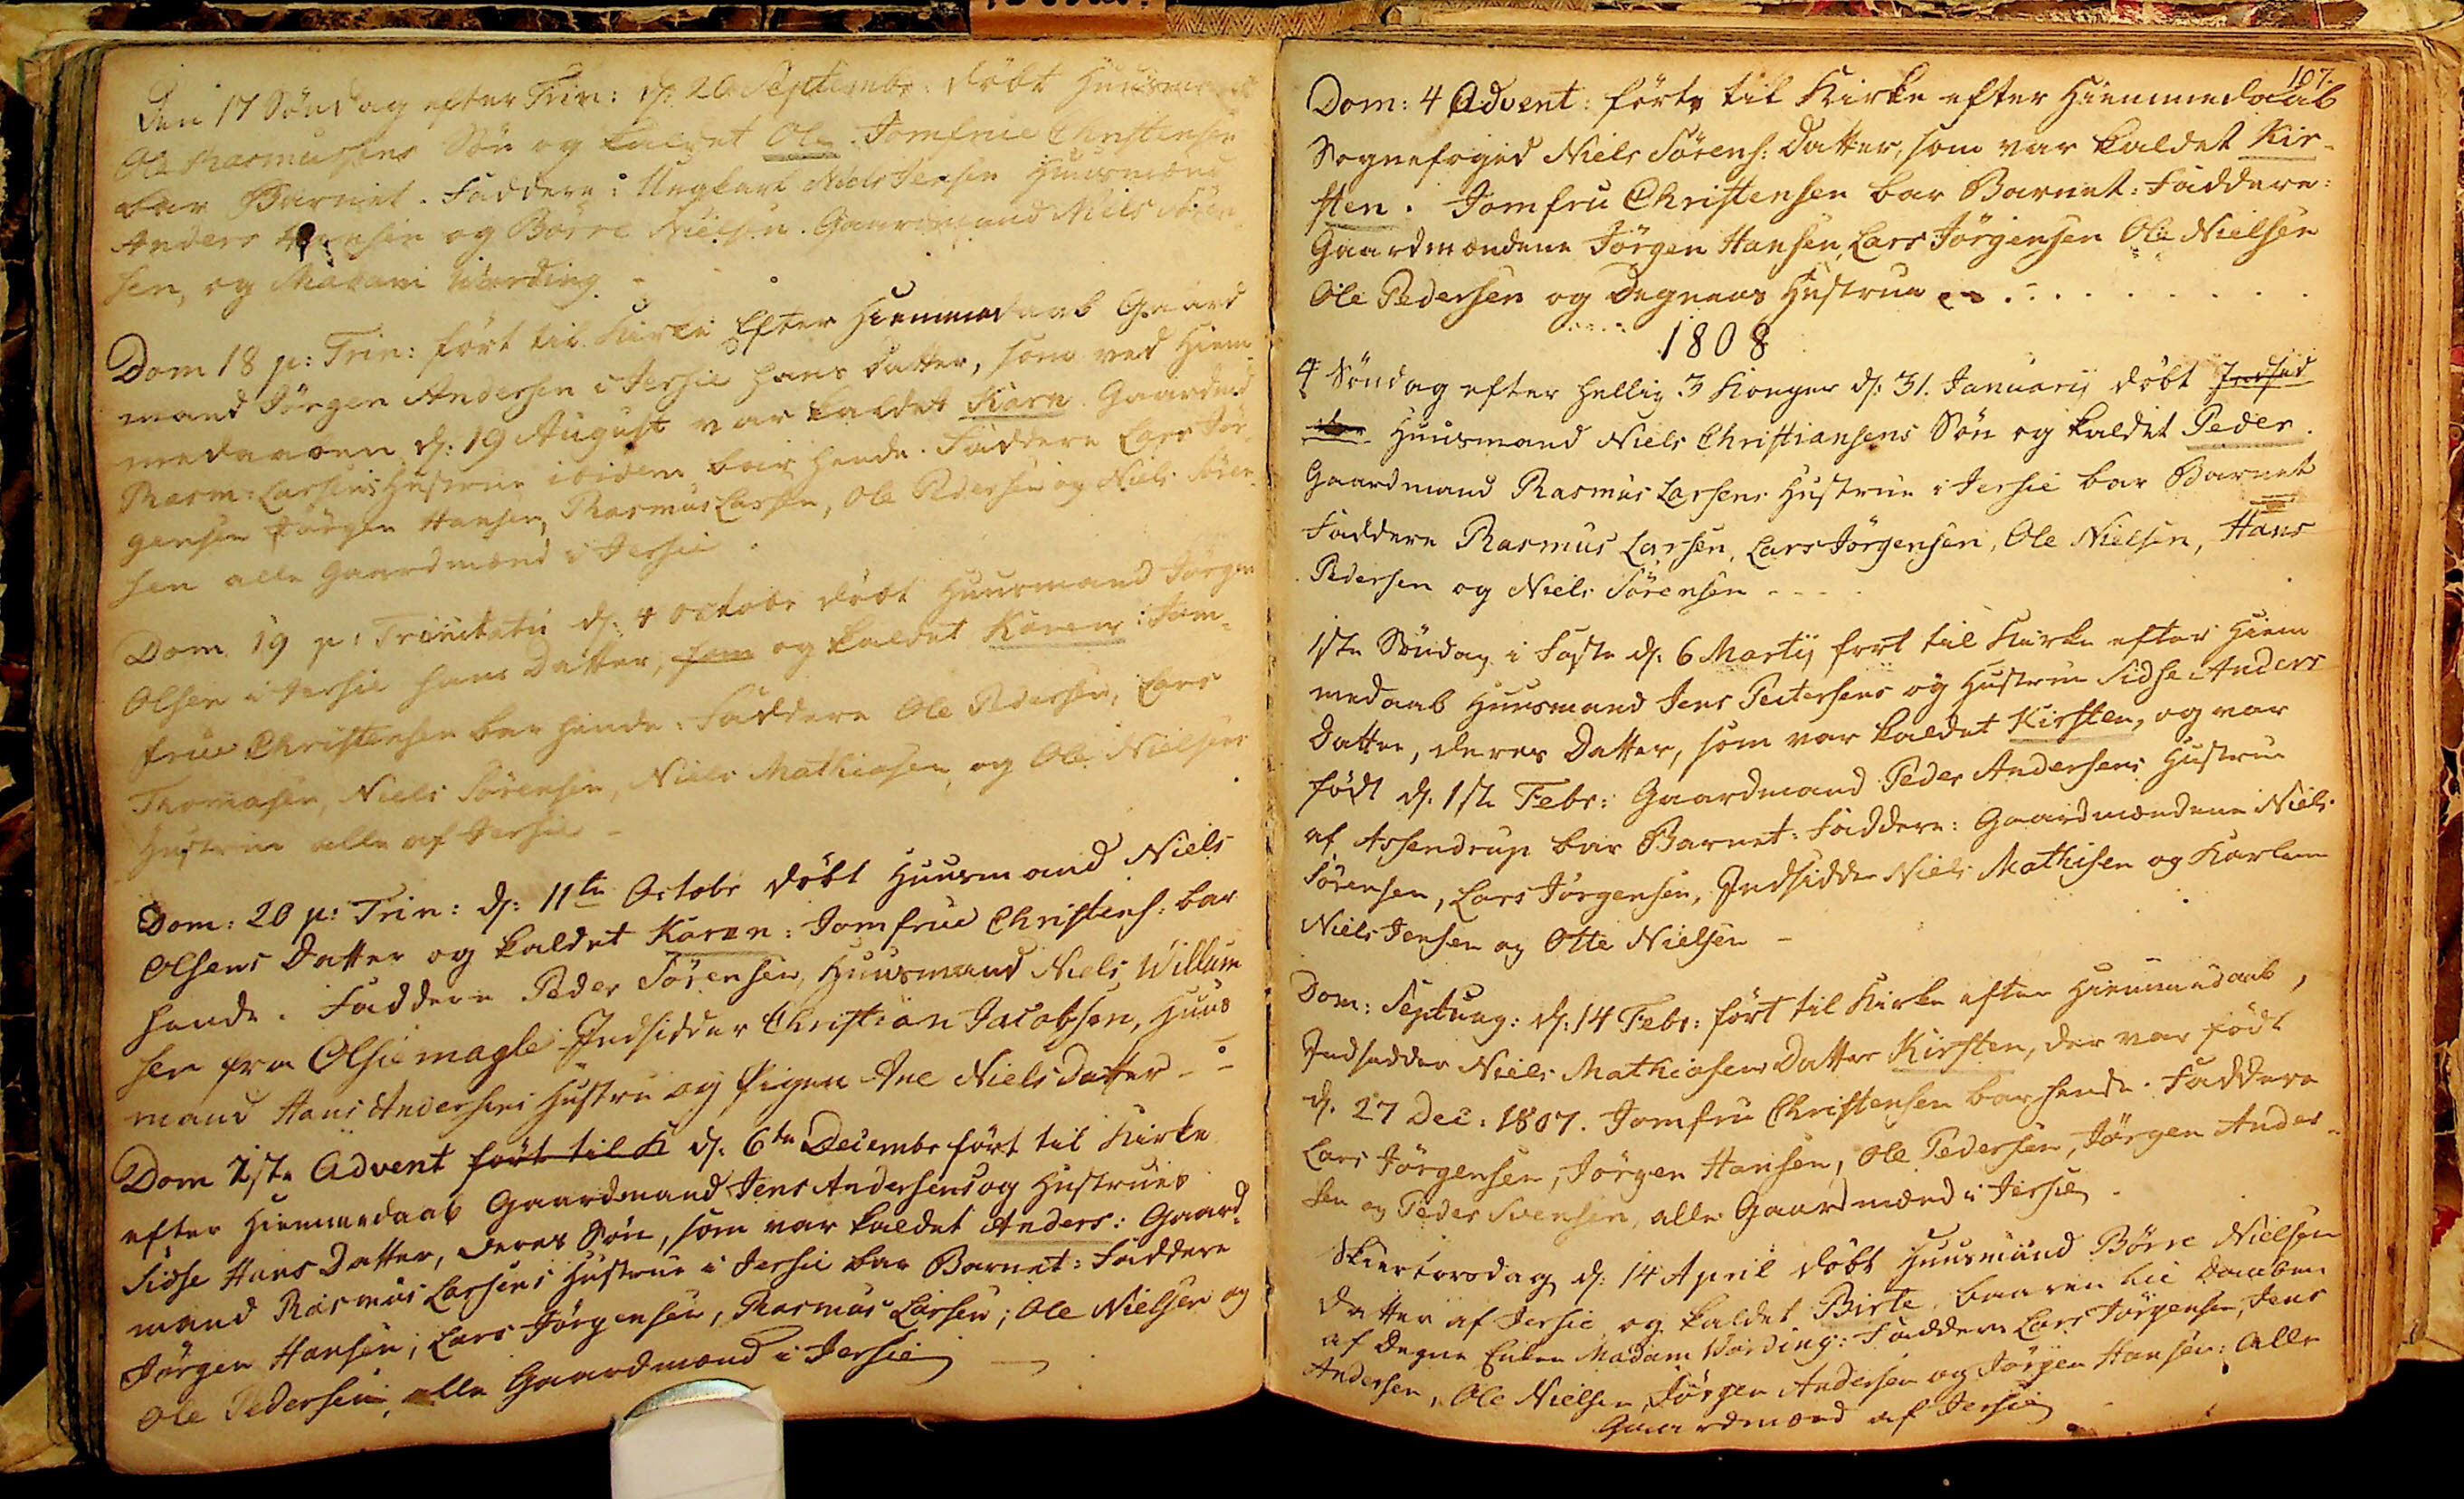Den 17 Søndag efter Trin: d: 20 Septembr: døbt Huusmand
Ole Rasmussens Søn og kaldet Ole. Jomfrue Christensen
bar Barnet. Faddere: Ungkarl Niels Jensen Huusmænd
Anders Hansen og Børre Nielsen. Gaardmand Niels Søren
sen, og Madam Wording.
Dom 18 p: Trin: ført til Kirke Efter Hiemmedaab Gaard
mand Jørgen Andersen i Jersie hans Datter, som ved Hiem
medaaben d: 19 August var kaldet Karn. Gaardmd
Rasm: Larsens Hustrue ibidem bar hende. Faddere Lars Jør
gensen, Jørgen Hansen, Rasmus Larsen, Ole Pedersen og Niels Søren
sen alle Gaardmænd i Jersie.
Dom. 19 p: Trinitatis d: 4 Octobr døbt Huusmand Jørgen
Olsen i Jersie hans Datter, som og kaldet Karen: Jom¬
frue Christensen bar hende: Faddere Ole Pedersen, Lars
Thomasen, Niels Sørensen, Niels Mathiasen, og Ole Nielsens
Hustrue alle af Jersie.
Dom: 20 p: Trin: d: 11te Octobr døbt Huusmand Niels
Olsens Datter og kaldet Karen: Jomfrue Christens: bar
hende. Faddere Peder Sørensen, Huusmand Niels Willum
sen fra Ølsiemagle Indsidder Christian Jacobsen, Huus
mand Hans Andersens Hustru og Pigen Ane Niels Datter_ _
Dom 2ste Advent ført til K d: 6te Decembr ført til Kirke
efter Hiemmedaab Gaadmand Jens Andersens og Hustrues
Sidse Hans Datter, deres Søn, som var kaldet Anders: Gaard¬
mand Rasmus Larsens Hustrue i Jersie bar Barnet: Faddere
Jørgen Hansen, Lars Jørgensen, Rasmus Larsen; Ole Nielsen og
Ole Pedersen, alle Gaardmænd i Jersie
107.
Dom: 4 Advent: ført til Kirke efter Hiemmedaab
Sognefoged Niels Sørens: Datter, som var kaldet Kir¬
sten. Jomfru Christensen bar Barnet: Faddere:
Gardmændene Jørgen Hansen, Lars Jørgensen Ole Nielsen
Ole Pedersen og Degnens Hustrue
1808
4 Søndag efter hellig 3 Konger d: 31. Januarij døbt Indsid
der Huusmand Niels Christiansens Søn og kalet Peder.
Gaardmand Rasmus Larsens Hustrue i Jersie bar Barnet
Faddere Rasmus Larsen, Lars Jørgensen, Ole Nielsen, Hans
Pedersen og Niels Sørensen
1ste Søndag i Faste d: 6 Martij ført til Kirke efter Hiem
medaab Huusmand Jens Peitersens og hustrue Sidse Anders
Datter, deres Datter, som var kaldet Kirsten, og var
født d: 1ste Febr: Gaardmand Peder Andersens Hustrue
af Assendrup bar Barnet: Faddere: Gaardmændene Niels
Sørensen, Lars Jørgensen, Indsidder Niels Mathisen og karlene
Niels Jensen og Otte Nielsen.
Dom: Septuag: d: 14 Febr: ført til Kirke efter Hiemmedaab,
Indsidder Niels Mathiasens Datter Kirsten, der var født
d. 27 Dec: 1807. Jomfru Christensen bar hende. Faddere
Lars Jørgensen, Jørgen Hansen, Ole Pedersen, Jørgen Ander¬
sen og Peder Svensen, alle Gaardmænd i Jersie.
Skiertorsdag d: 14 April døbt Huusmand Børre Nielsens
Datter af Jersie og kaldet Birte, baaren til Daaben
af Degne Enken Madam Wording: Faddere: Lars Jørgensen, Jens
Andersen, Ole Nielsen, Jørgen Andersen og Jørgen Hansen: Alle
Gaardmænd af Jersie.
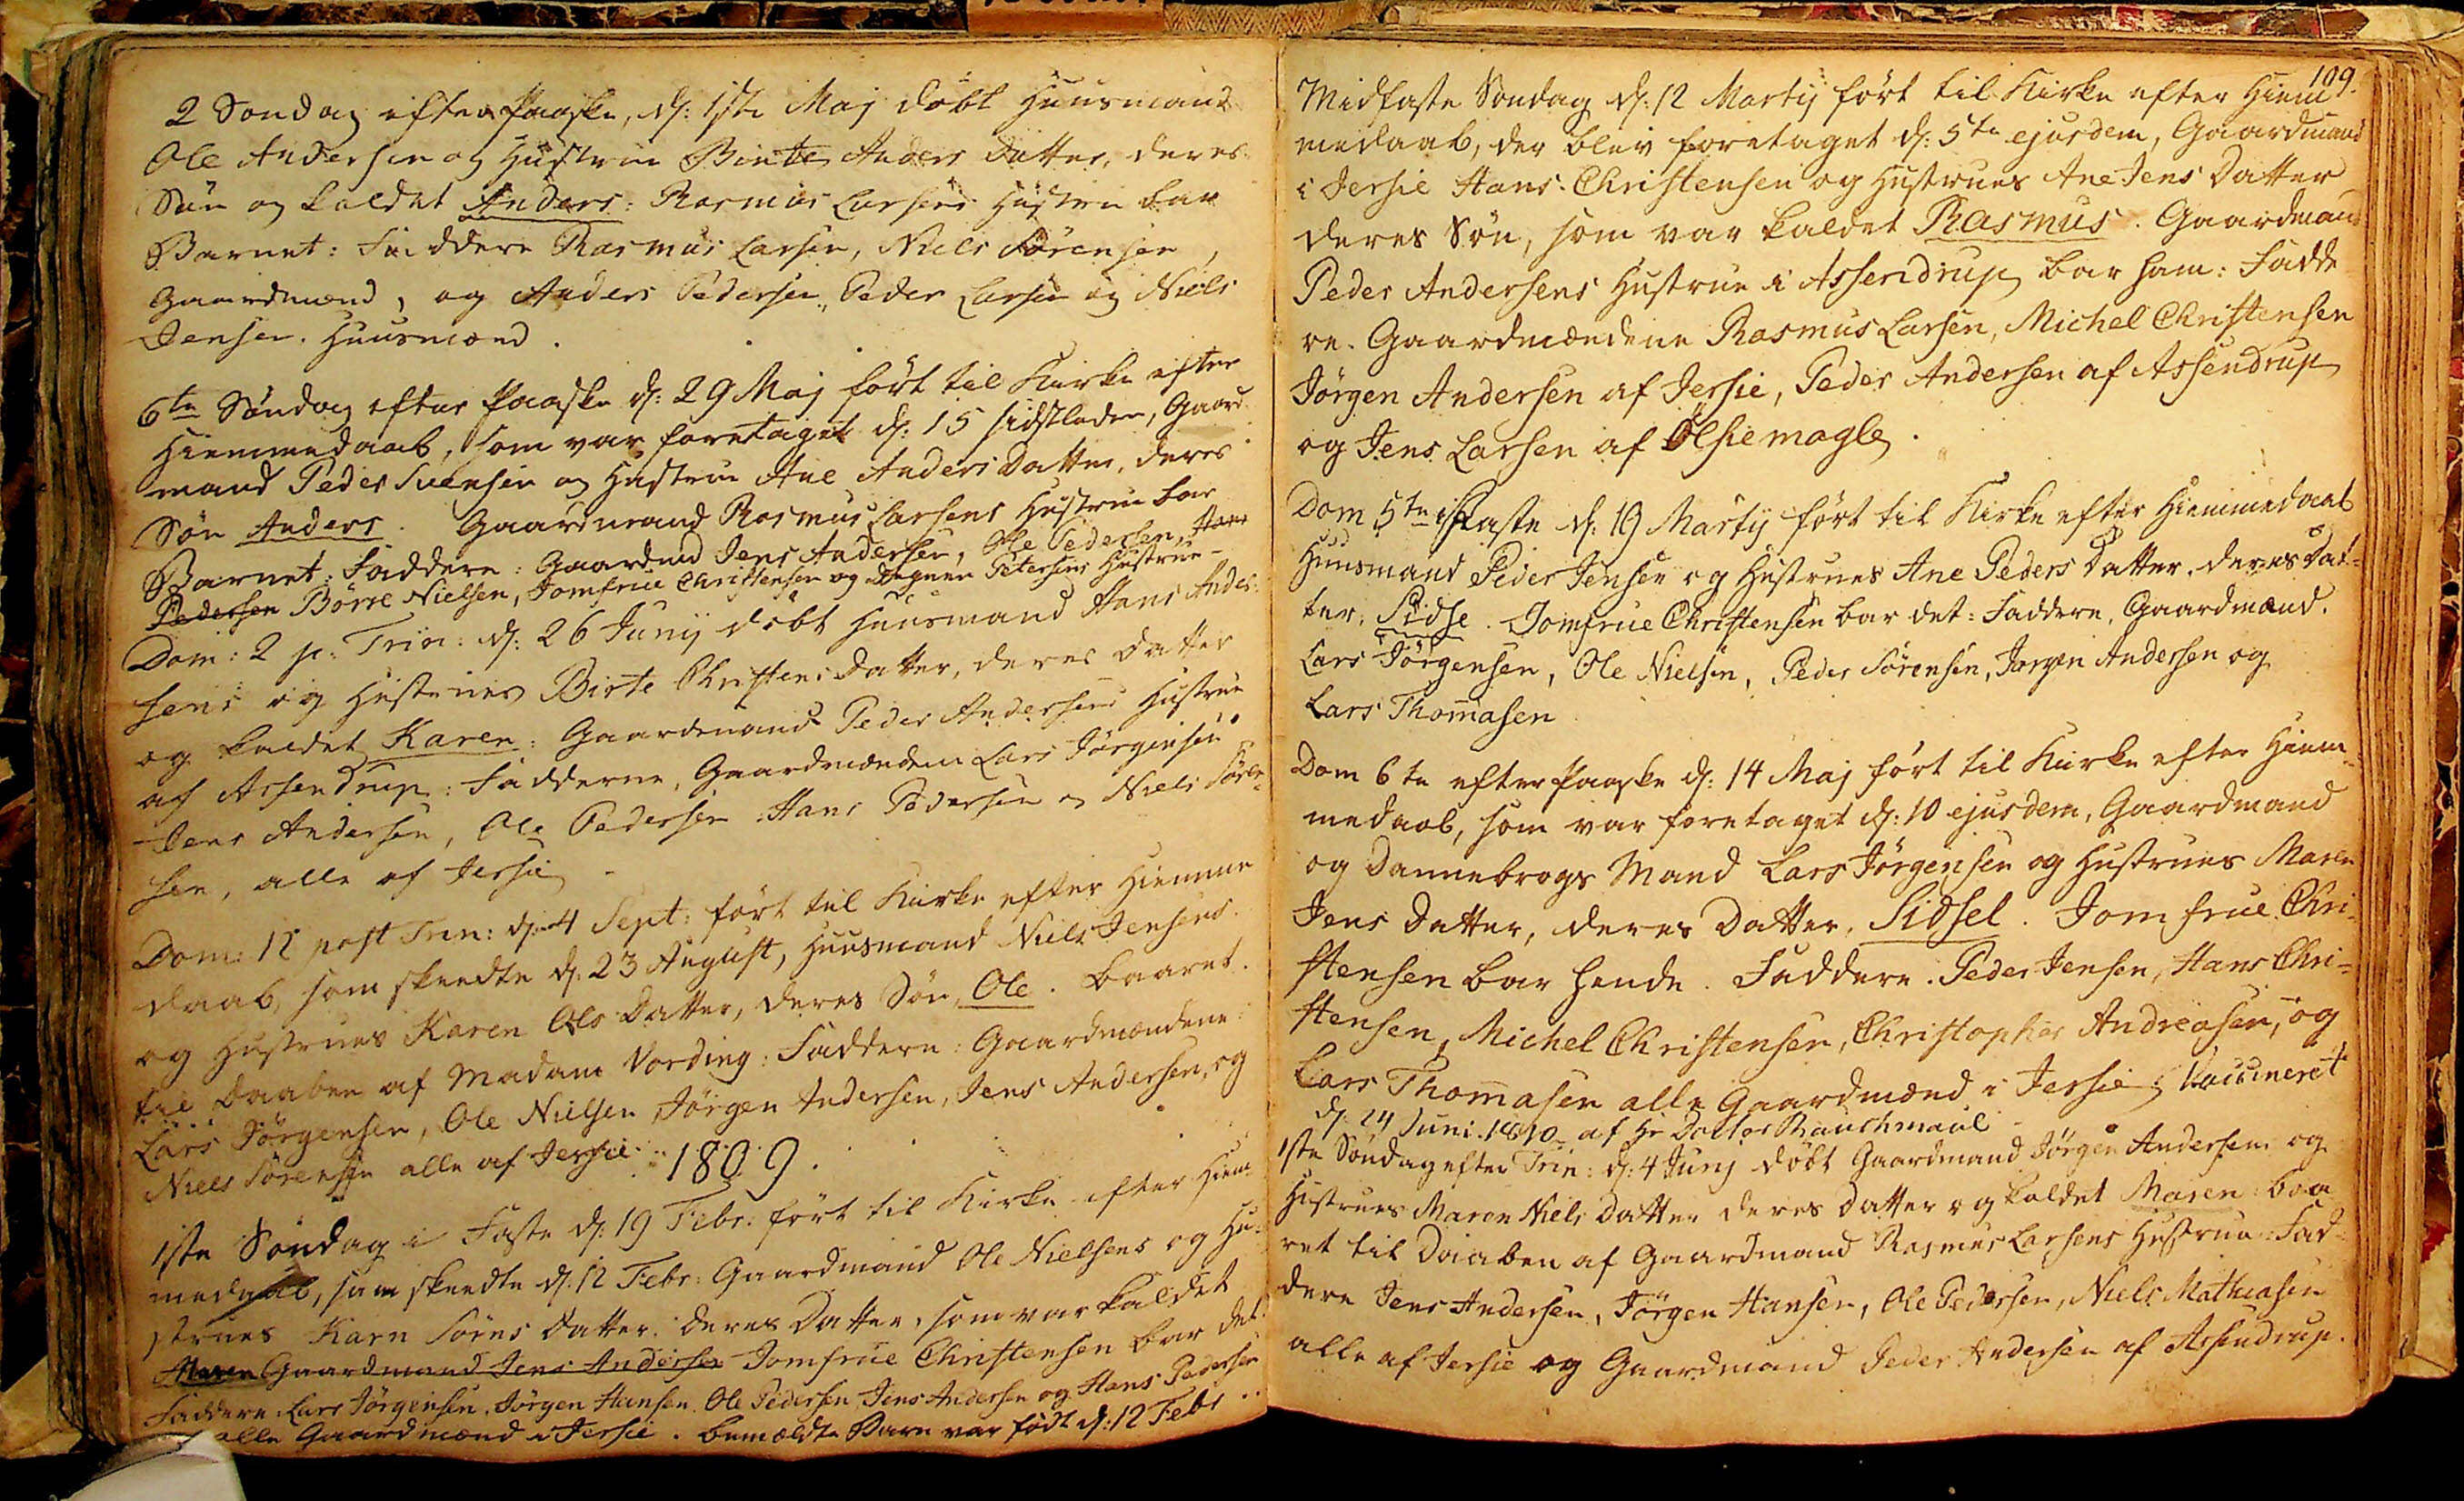2 Søndag efter Paaske, d: 1ste Maj døbt Huusmand
Ole Andersen og Hustrue Birte Anders Datter, deres
Søn og kaldet Anders: Rasmus Larsens Hustrue bar
Barnet: Faddere Rasmus Larsen, Niels Sørensen,
Gaardmænd, og Anders Pedersen, Peder Larsen og Niels
Jensen, Huusmænd.
6te Søndag efter Paaske d: 29 Maj ført til Kirke efter
Hiemmedaab, som var foretaget d: 15 sidstleden, Gaard
mand Peder Svensen og Hustrue Ane Anders Datter, deres
Søn Anders. Gaardmand Rasmus Larsens Hustrue bar
Barnet: Faddere: Gaardmd Jens Andersen, Ole Pedersen, Hans 
Pedersen Børre Nielsen, Jomfrue Christensen og Degnen Petersens Hustrue -
Dom: 2. p: Trin: d: 26 Junij døbt Huusmand Hans Ander
sens og Hustrues Birte Christens Datter, deres Datter
og kaldet Karen: Gaardmand Peder Andersens Hustrue
af Assendrup: Fadderne, Gaardmænden Lars Jørgensen
Jens Andersen, Ole Pedersen, Hans Pedersen og Niels Søren¬
sen, alle af Jersie.
Dom: 12 post Trin: d: 4 Sept: ført til Kirke efter Hiemme
daab, som skeedte d: 23 August, Huusmand Niels Jensens
og Hustrues Karen Ols Datter, deres Søn, Ole. Baaret
til Daaben af Madam Vording: Faddere: Gaardmændene:
Lars Jørgensen, Ole Nielsen, Jørgen Andersen, Jens Andersen og
Niels Sørensen alle af Jersie.
1809.
1ste Søndag i Faste d: 19 Febr: ført til Kirke efter Hiem
medaab, som skeedte d: 12 Febr: Gaardmand Ole Nielsens og Hu¬
strues Karn Sørns Datter, Deres Datter, som var kaldet
Maren Gaardmand Jens Andersen Jomfrue Christensen bar det.
Faddere: Lars Jørgensen, Jørgen Hansen, Ole Pedersen, Jens Andersen og Hans Pedersen
alle Gaardmænd i Jersie. Bemældte Barn var født d: 12 Febr.
109.
Midfaste Søndag d: 12 Martij ført til Kirke efter Hiem
medaab, der blev foretaget d: 5te ejusdem, Gaardmand
i Jersie Hans Christensen og Hustrues Ane Jens Datter
deres Søn, som var kaldet Rasmus. Gaardmand
Peder Andersens Hustrue i Assendrup bar ham: Fadde
re. Gaardmændene Rasmus Larsen, Michel Christensen
Jørgen Andersen af Jersie, Peder Andersen af Assendrup
og Jens Larsen af Ølsiemagle.
Dom 5te i Faste d: 19 Martij ført til Kirke efter Hiemmedaab
Huusmand Peder Jensen og Hustrues Ane Peders Datter, deres Dat¬
ter, Sidse. Jomfrue Christensen bar det: Faddere. Gaardmænd.
Lars Jørgensen, Ole Nielsen, Peder Sørensen, Jorgen Andersen og
Lars Thomasen.
Dom 6te efter Paaske d: 14 Maj ført til Kirke efter Hiem
medaab, som var foretaget d: 10 ejusdem, Gaardmand
og Dannebrogs Mand Lars Jørgensen og Hustrues Maren
Jens Datter, deres Datter, Sidsel. Jomfrue Chri¬
stensen bar hende. Faddere. Peder Jensen, Hans Chri¬
stensen, Michel Christensen, Christopher Andreasen, og
Lars Thomasen alle Gaardmænd i Jersie. Vaccineret
d: 24 Juni. 1810 af Hr Doctor Bauchmaul.
1ste Søndag efter Trin: d: 4 Junj døbt Gaardmand Jørgen Andersen og
Hustrues Maren Niels Datter deres Datter og kaldet Maren: Baa
ret til Daaben af Gaardmand Rasmus Larsens Hustrue: Fad¬
dere Jens Andersen, Jørgen Hansen, Ole Pedersen, Niels Mathiasen
alle af Jersie og Gaardmand Peder Andersen af Assendrup.